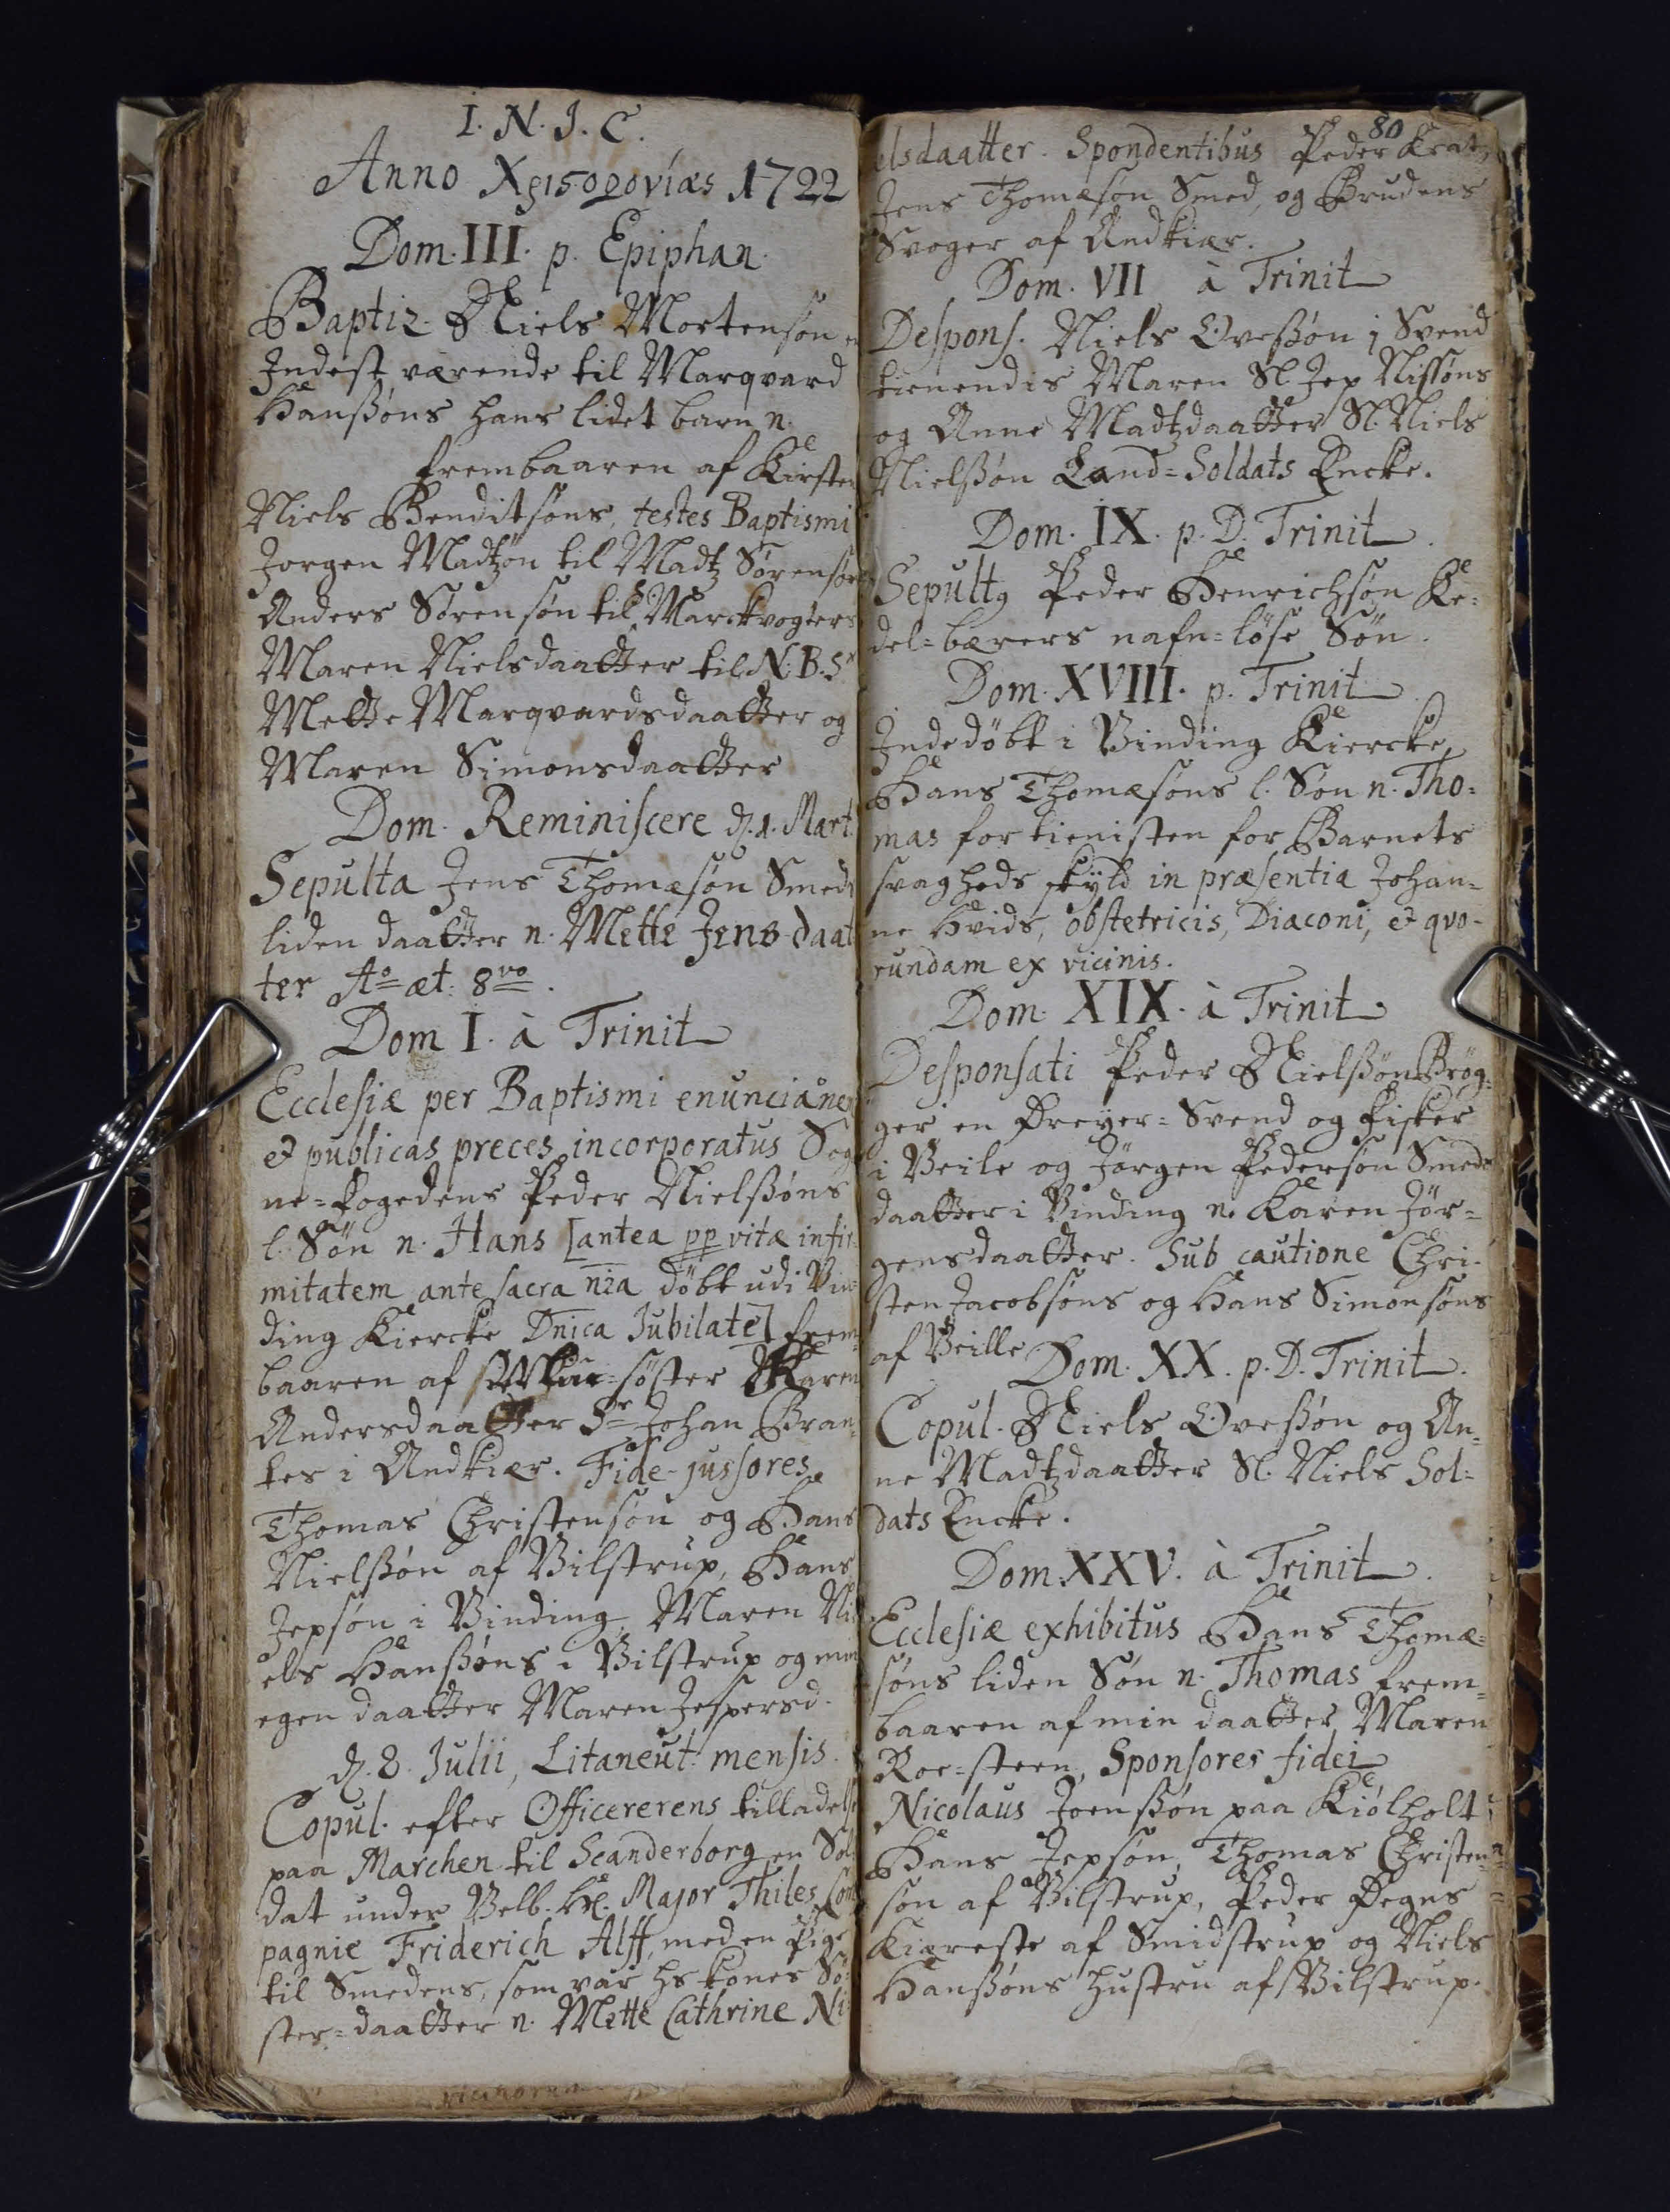I. N. J. C.
Anno Xq##ovias 1722
Dom. III. p. Epiphan.
Baptiz. Niels Mortensøn en
Indest værende til Marqvard
Hanssøns hans lidet barn n.
frembaaren af Kirsten
Niels Benditsøns. testes Baptismi
Jørgen Madtzøn til Madtz Sørensøn
Anders Sørensøn til Marckvogters
Maren Nielsdaatter til N.B.S
Mette Marqvardsdaatter og
Maren Simonsdaatter.
Dom. Reminiscere d. 1. Mart.
Sepulta Jens Thomæsøn Smeds
liden daatter n. Mette Jens-daat
ter Ao æt: 8vo
Dom I. a Trinit
Ecclesiæ per Baptismi enuncianem 
et publicas preces incorporatus Sog
ne=Fogedens Peder Nielssøns
l. Søn n. Hans [antea pp vitæ infir¬
mitatem ante sacra nia døbt udi Vin¬
ding Kiercke Dnica Jubilate] frem¬
baaren af Min=søster Karen
Andersdaatter Sr Johan Bran¬
tes i Andkiær. Fide-jussores
Thomas Christensøn og Hans
Nielssøn af Vilstrup, Hans
Jepsøn i Vinding, Maren Ni
els Hanssøns i Vilstrup og min
egen daatter Maren Jespersd.
d. 8. Julii, Litaneut. mensis
Copul. efter Officererens tilladelse
paa Marchen til Scanderborg en Sol
dat under Velb. Hl. Major Thiles Com
pagnie Friderich Alff, med en Pige
til Smedens, som var hs. kones Sø¬
ster=daatter n. Mette Cathrine Ni
80
elsdaatter. Spondentibus Peder Krat,
Jens Thomæsøn Smed, og Brudens
Svoger af Andkiær.
Dom. VII a Trinit
Sespons. Niels Ovessøn 1 Svend
tienendis Maren Sl. Jep Nissøns
og Anna Madtzdaatter Sl. Niels
Nielssøn Land=Soldats Encke.
Dom. IX p. D. Trinit
Sepultq Peder Henrichsøn Ke¬
del=bærers nafn=løse Søn.
Dom. XVIII. p. Trinit
Indedøbt i Vinding Kiercke 
Hans Thomæsøns l. Søn n. Tho¬
mas for tienisten for Barnets
svagheds skyld in præsentia Johan¬
ne Hvids, obstetricis, Diaconi, et qvo¬
rundam ex vicinis.
Dom. XIX. a Trinit
Desponsati Peder Nielssøn Brøg¬
ger en Dreyer=Svend og Fisker
i Veile og Jørgen Pedersøn Smeds
daatter i Vinding n. Karen Jør
gensdaatter. Sub cautione Chri¬
sten Jacobsøns og Hans Simonsøns
af Veille
Dom. XX. p. D. Trinit
Copul. Niels Ovessøn og An¬
ne Madtzdaatter Sl. Niels Sol¬
dats Encke.
Dom XXV. a Trinit.
Ecclesiæ exhibitus Hans Thomæ¬
søns liden Søn n. Thomas, frem¬
baaren af min daatter Maren
Roe=steen, Sponsores fidei
Nicolaus Joenssøn paa Kiølholt
Hans Jepsøn, Thomas Christen¬
søn af Vilstrup, Peder Degns
Kiæreste af Smidstrup og Niels
Hanssøns hustru af Vilstrup.